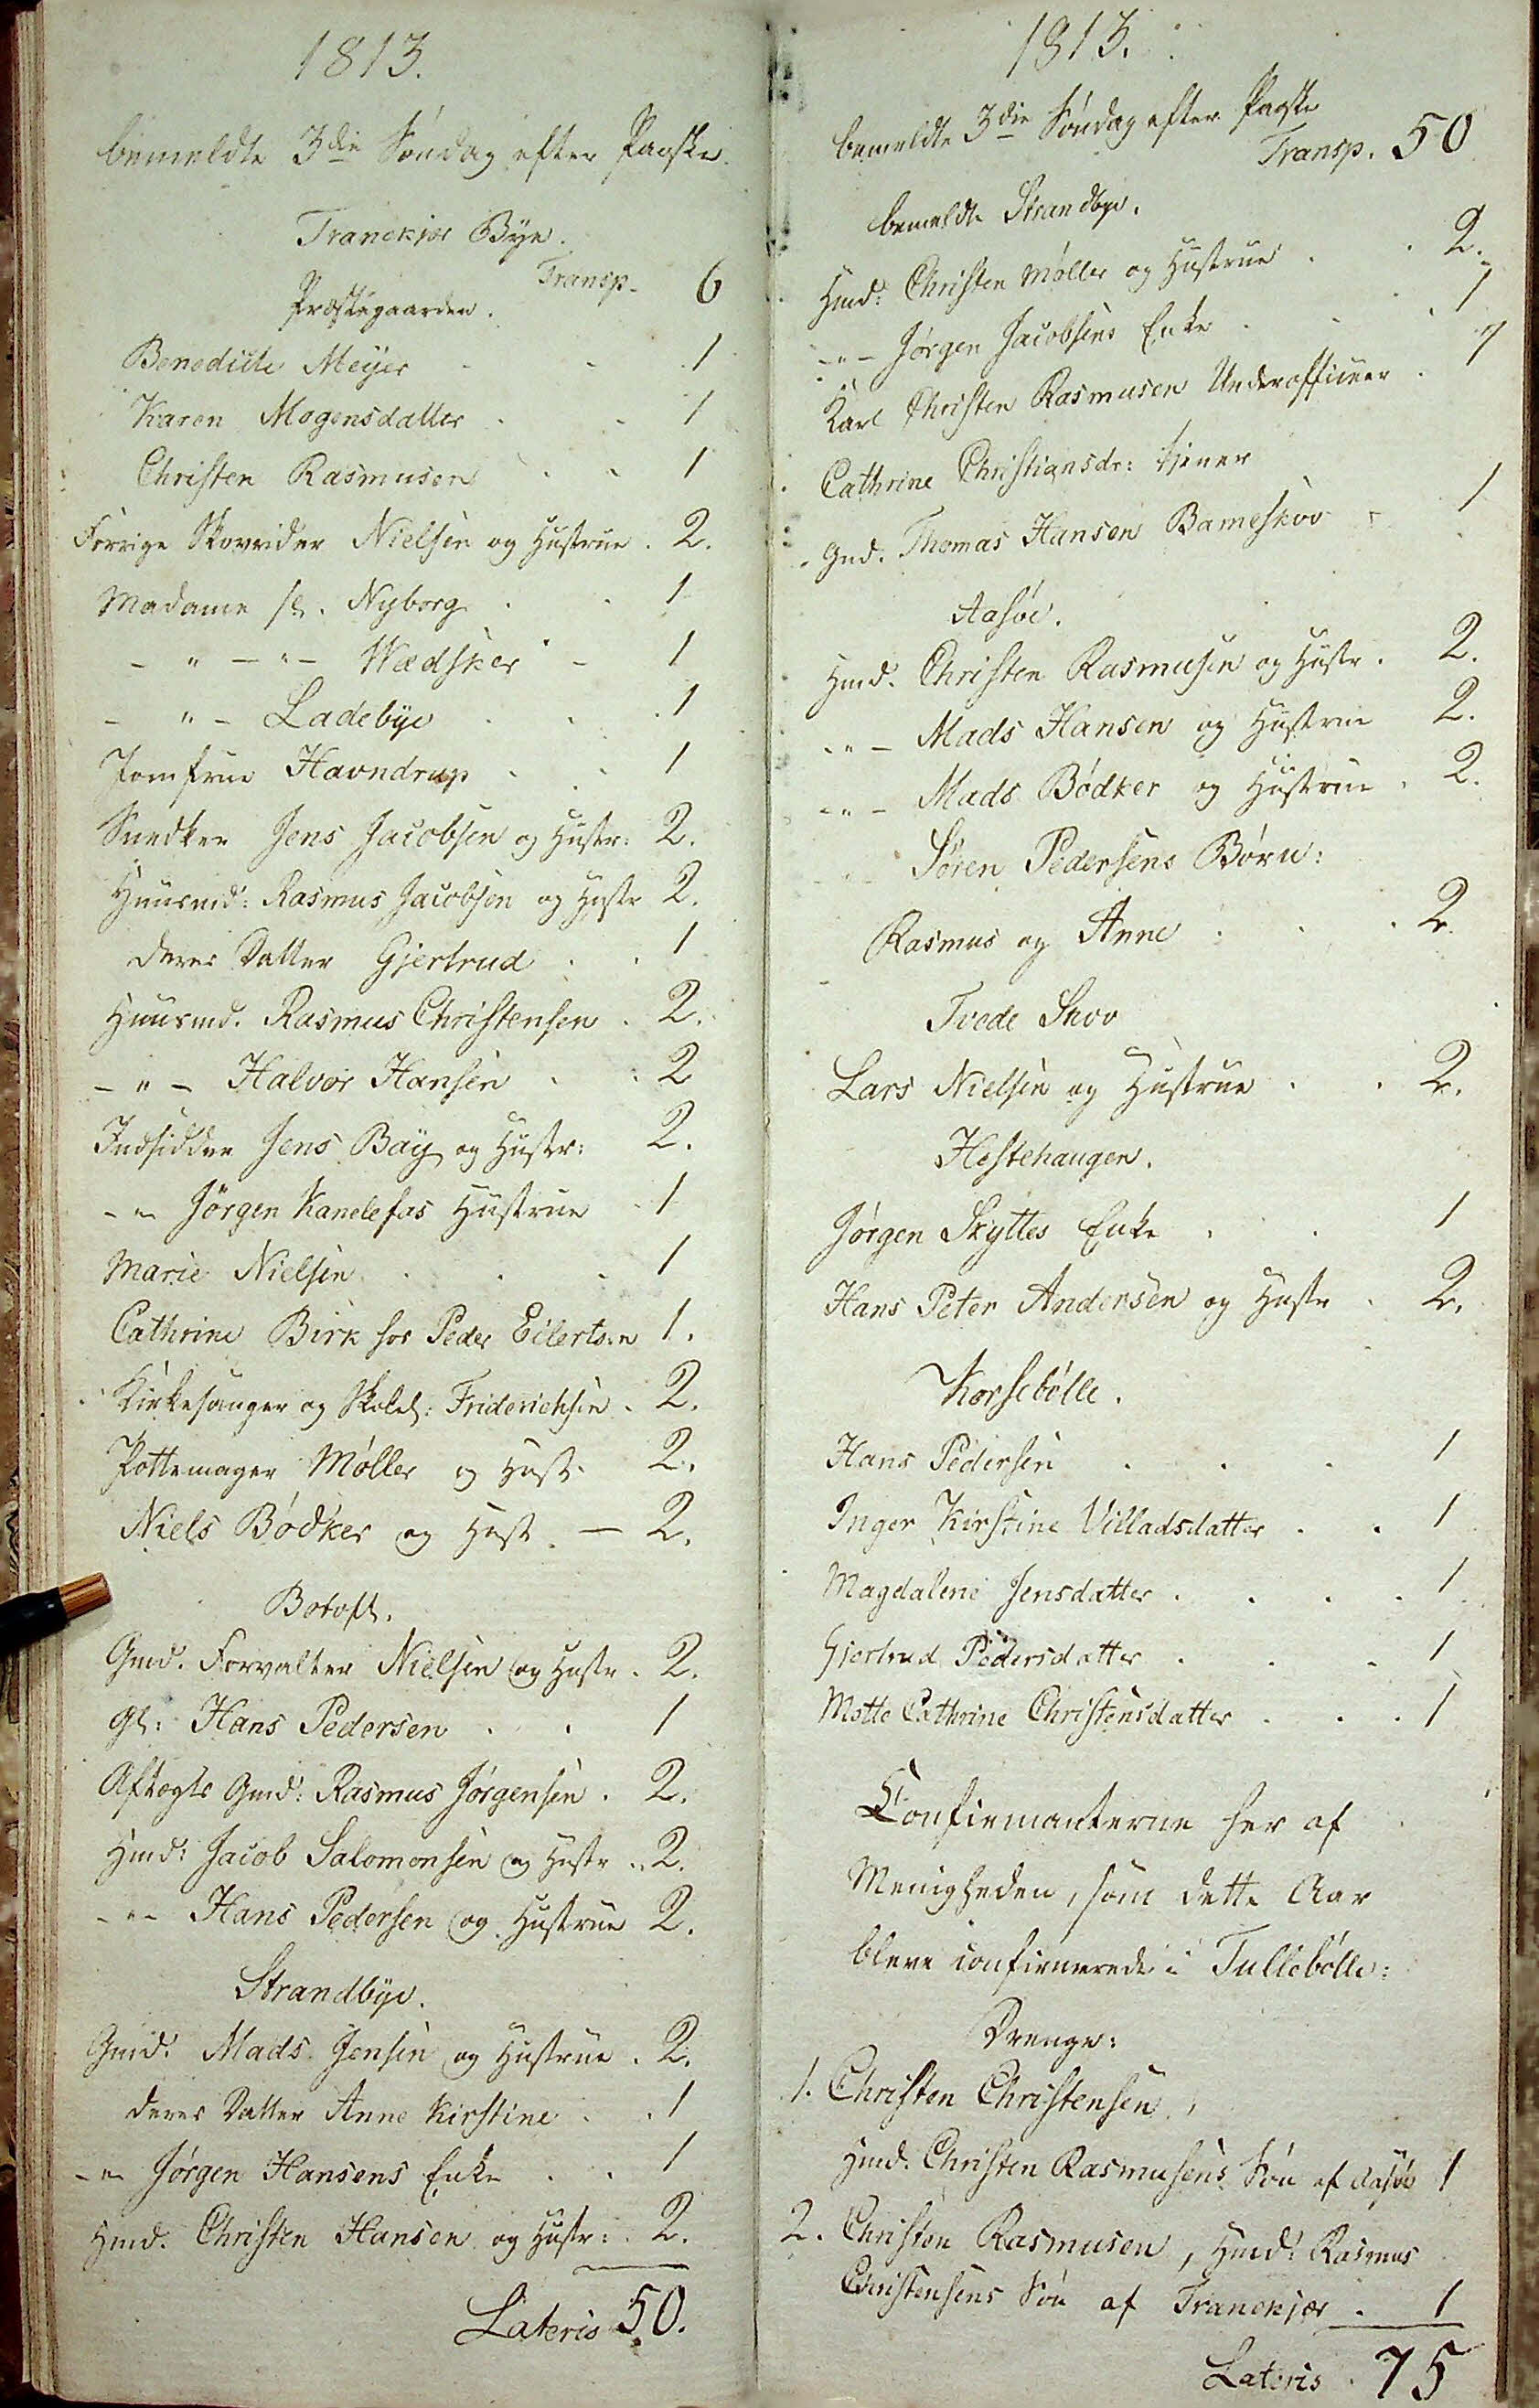1813.
bemeldte 3die Søndag efter Paaske.
Tranekjær Bye.
Præstegaarden.
Transp. 6
Benedicte Meyer . . . 1
Karen Mogensdatter . . 1
Christen Rasmusen . . 1
Forrige Skovrider Nielsen og Hustrue . 2.
Madame Sl. Nyborg . . 1
-"-"- Vædsker . - 1
-"-  Ladebye . . . 1
Jomfrue Havndrup . . 1
Snedker Jens Jacobsen og Hustr : 2.
Huusmd: Rasmus Jacobsen og Hustr 2.
deres Datter Gjertrud . . 1
Huusmd. Rasmus Christensen . 2.
-"- Halvor Hansen .  : 2
Indsidder Jens Bay og Hustr : 2.
-"- Jørgen Kanelefas Hustrue . 1
Marie Nielsen. . . . 1
Cathrine Birk hos Peder Eilertsen 1.
Kirkesanger og Skolel: Friderichsen . 2.
Pottemager Møller og Hust . 2.
Niels Bødker og Hust . - 2.
Botoft.
Gmd. Forvalter Nielsen og Hustr . 2.
Gl: Hans Pedersen . . 1
Aftægts Gmd: Rasmus Jørgensen . 2.
Hmd: Jacob Salomonsen og Hustr . . 2.
-"- Hans Pedersen og Hustrue 2.
Strandbye.
Gmd. Mads Jensen og Hustrue . 2.
deres Datter Anne Kirstine . . 1
-"- Jørgen Hansens Enke . . 1
Hmd. Christen Hansen og Hustr : . 2.
Lateris 50.
1813.
bemeldte 3die Søndag efter Paaske.
Transp. 50
bemeldte Strandbye.
Hmd Christen Møller og Hustrue . . 2.
-"- Jørgen Jacobsens Enke . . . . 1
Karl Christen Rasmusen Underofficeer . 1
Cathrine Christiansdr: tjener
Gnd. Thomas Hansen Bameskov - 1
Aasøe.
Hmd. Christen Rasmusen og Hustr . 2.
-"- Mads Hansen og Hustrue 2.
-"- Mads Bødker og Hustrue . 2.
Søren Pedersens Børn:
Rasmus og Anne . . . 2.
Tvede Skov
Lars Nielsen og Hustrue . . 2.
Hestehaugen.
Jørgen Skyttes Enke . . 1
Hans Peter Andersen og Hustr . 2.
Korsebølle.
Hans Pedersen . . . 1
Inger Kirstine Villadsdatter . . 1
Magdalene Jensdatter . . . . 1
Gjertrud Pedersdatter . . . 1
Mette Cathrine Christensdatter . . . 1
Confirmaterne her af
Menigheden, som dette Aar
bleve confirmerede i Tullebølle:
Drenge:
1. Christen Christensen
Hmd. Christen Rasmusens Søn af Aasøe 1
2. Christen Rasmusen, Hmd: Rasmus
Christensens Søn af Tranekjær . 1
Lateris . 75
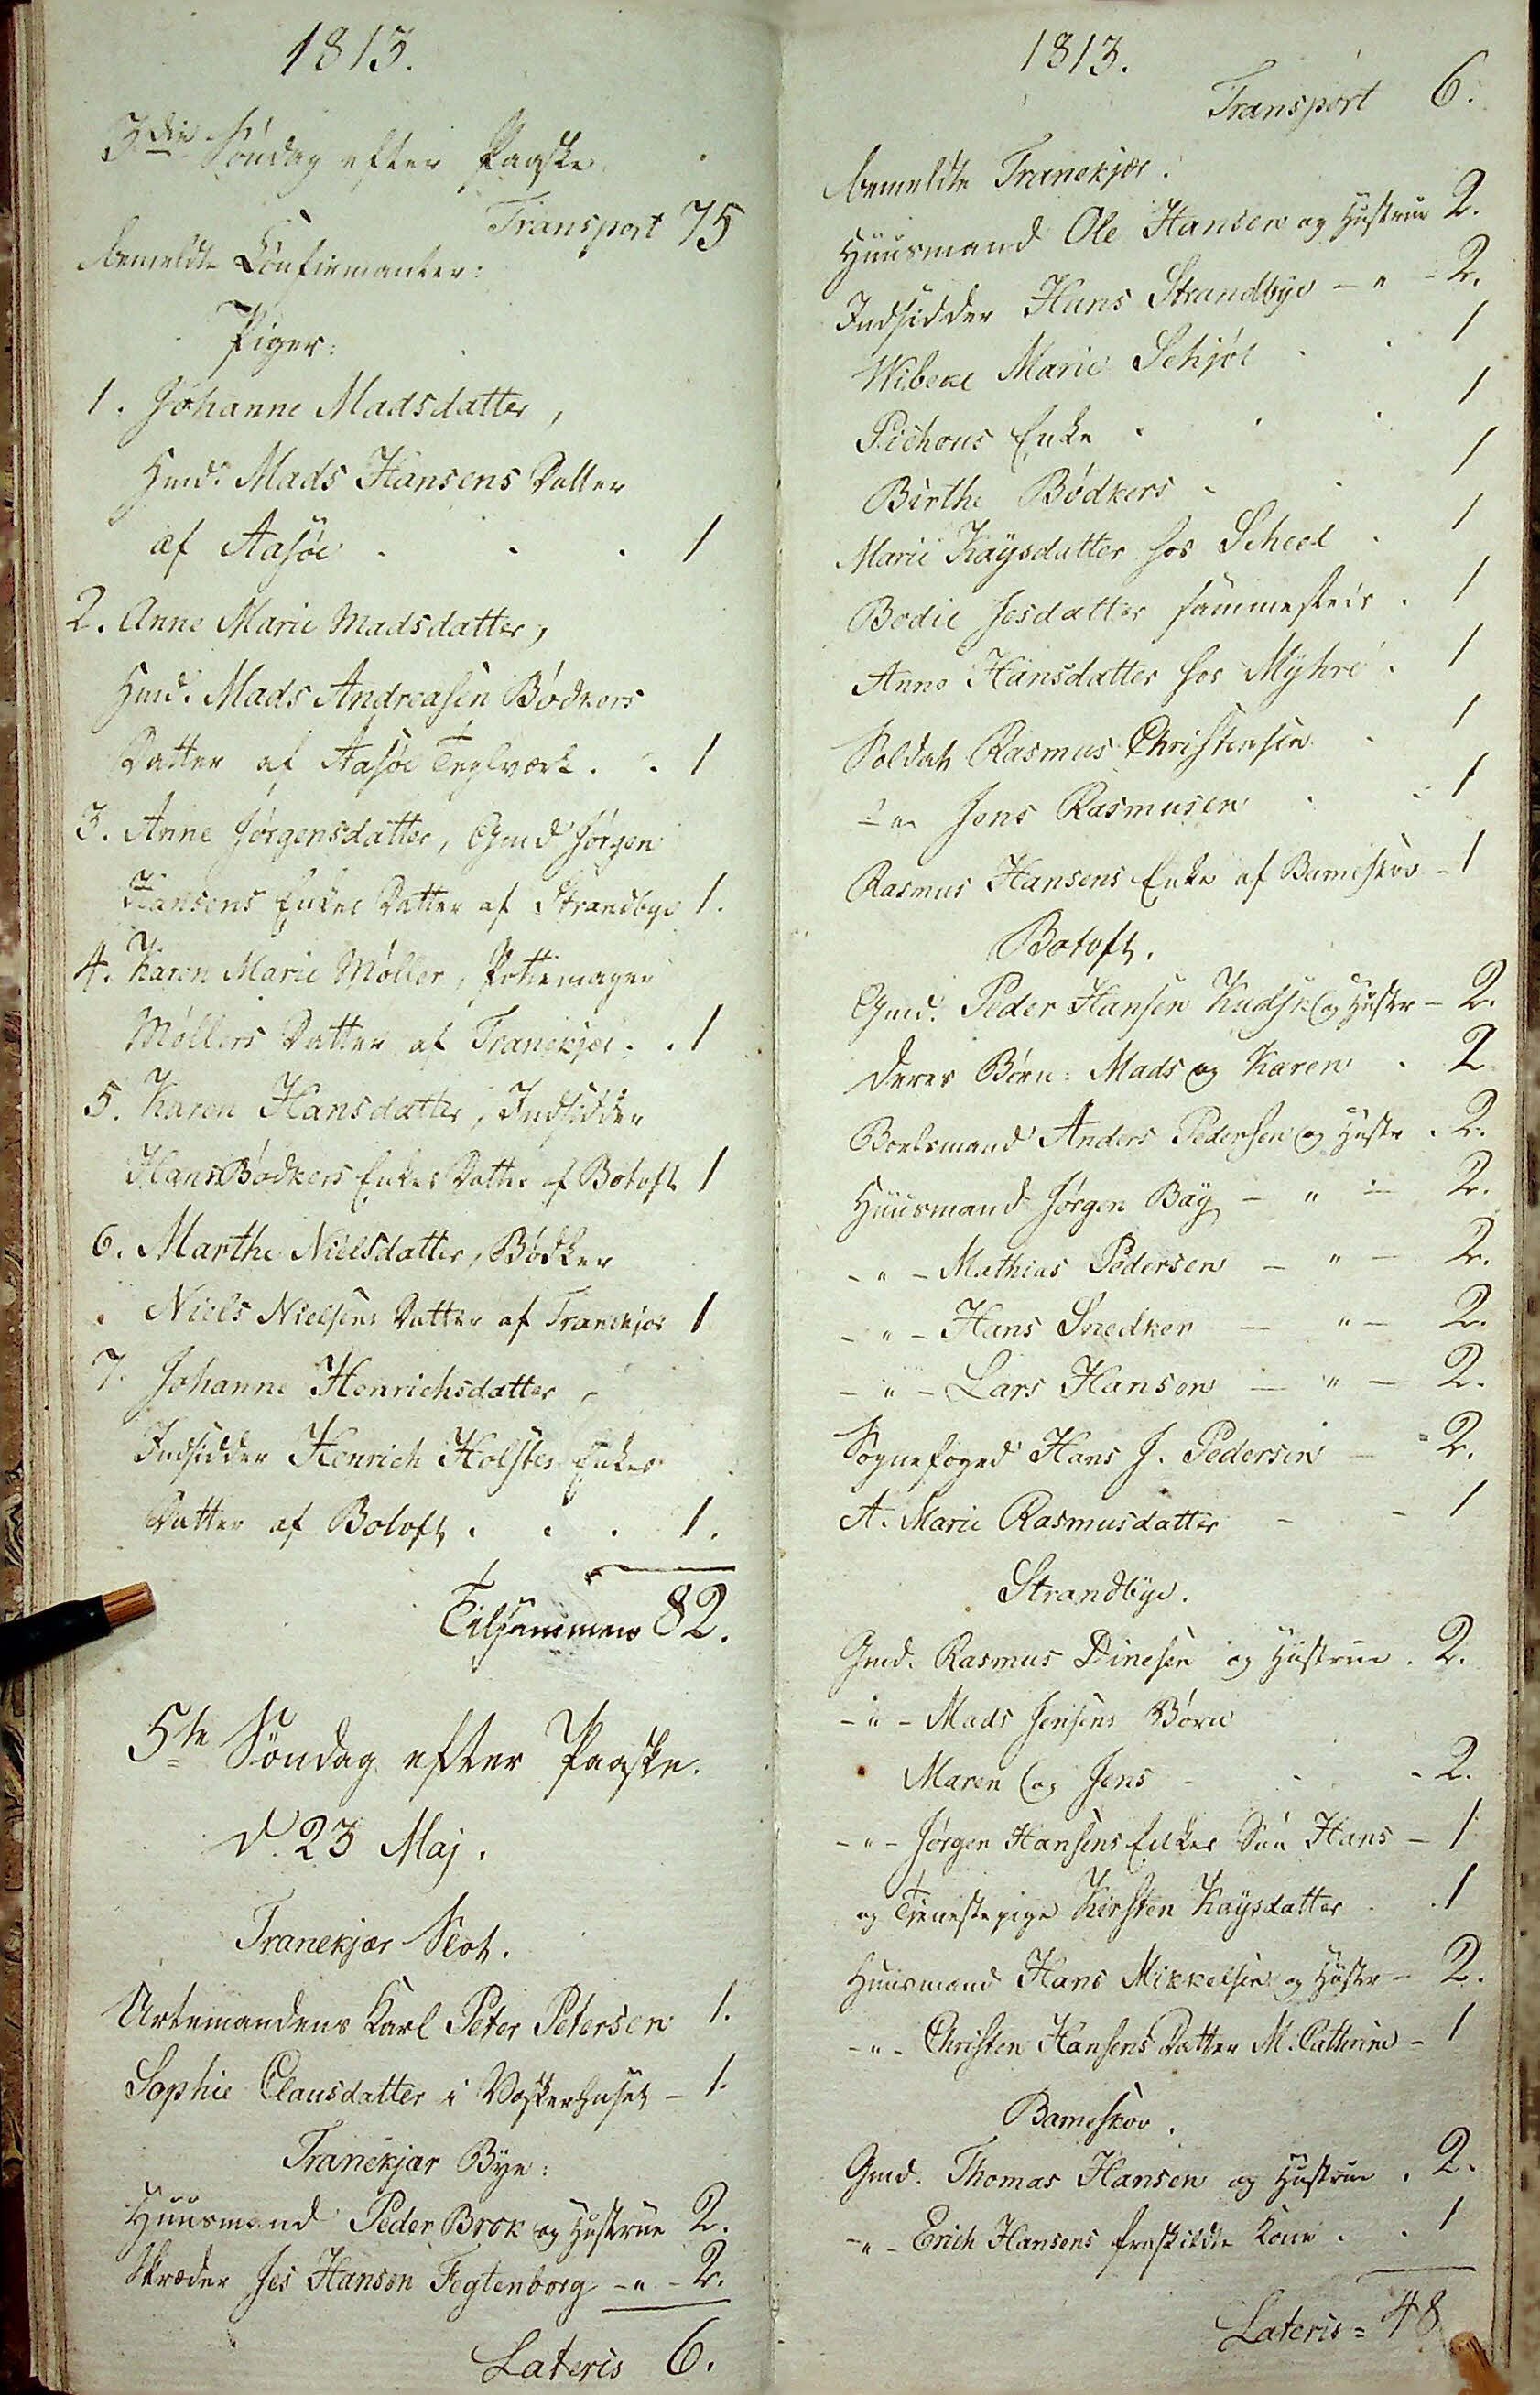1813.
3die Søndag efter Paaske
Transport 75
bemeldte Confirmanter:
Piger:
1. Johanne Madsdatter
Hmd. Mads Hansens Datter
af Aasøe . . . 1
2. Anne Marie Madsdatter.
Hmd. Mads Andreasen Bødkers
Datter af Aasøe Teglværk . . 1
3. Anne Jørgensdatter, Gmd Jørgen
Hansens Enkes Datter af Strandbye 1.
4. Karen Marie Møller, Pottemager
Møllers Datter af Tranekjær . . 1
5. Karen Hansdatter, Indsidder
Hans Bødkers Enkes Datter af Botoft 1
6. Marthe Nielsdatter, Bødter
Niels Nielsens Datter af Tranekjær 1
7. Johanne Henrichsdatter
Indsidder Henrich Holstes Enkes
Datter af Botoft . . . 1.
Tilsammen 82.
5te Søndag efter Paaske.
d. 23 Maj.
Tranekjær Slot.
Urtemandens Karl Peter Petersen 1.
Sophie Clausdatter i Vaskenhuset  - 1.
Tranekjær Bye:
Huusmand Peder Brok og Hustrue 2.
Skræder Jes Hansen Fegtenborg -"- 2.
Lateris 6.
1813.
Transport 6.
bemeldte Tranekjær.
Huusmand Ole Hansen og Hustrue 2.
Indsidder Hans Strandbye -"- 2.
Wibene Marie Schjøt . . 1
Pichous Enke . . . 1
Birthe Bødkers  . . 1
Marie Kaysdatter hos Scheel . 1
Bodil Jesdatter sammesteds . 1
Anne Hansdatter hos Myhre . 1
Soldat Rasmus Christensen . 1
-"- Jens Rasmusen . . 1
Rasmus Hansens Enke af Bameskov - 1
Botoft.
Gmd. Peder Hansen Kudsk og Hustr - 2.
deres Børn: Mads og Karen . 2
Boelsmand Anders Pedersen og Hustr . 2.
Huusmand Jørgen Bay -"- 2.
-"- Mathias Pedersen -"- 2.
-"- Hans Snedker -"- 2.
-"- Lars Hansen -"- 2.
Sognefoged Hans J. Pedersens - = 2.
A. Marie Rasmusdatter - - 1
Strandbye.
Gmd. Rasmus Dinesen og Hustrue . 2.
-"- Mads Jensens Børn
Maren og Jens . . . 2.
-"- Jørgen Hansens Enkes Søn Hans - 1
og Tjenestepige Kirsten Hansdatter . . 1
Huusmand Hans Mikkelsens og Hustr - 2.
-"- Christen Hansens Datter M. Cathrine - 1
Bameskov.
Gmd. Thomas Hansen og Hustrue . 2.
-"- Erich Hansens fraskilte Kone . . 1
Lateris = 48